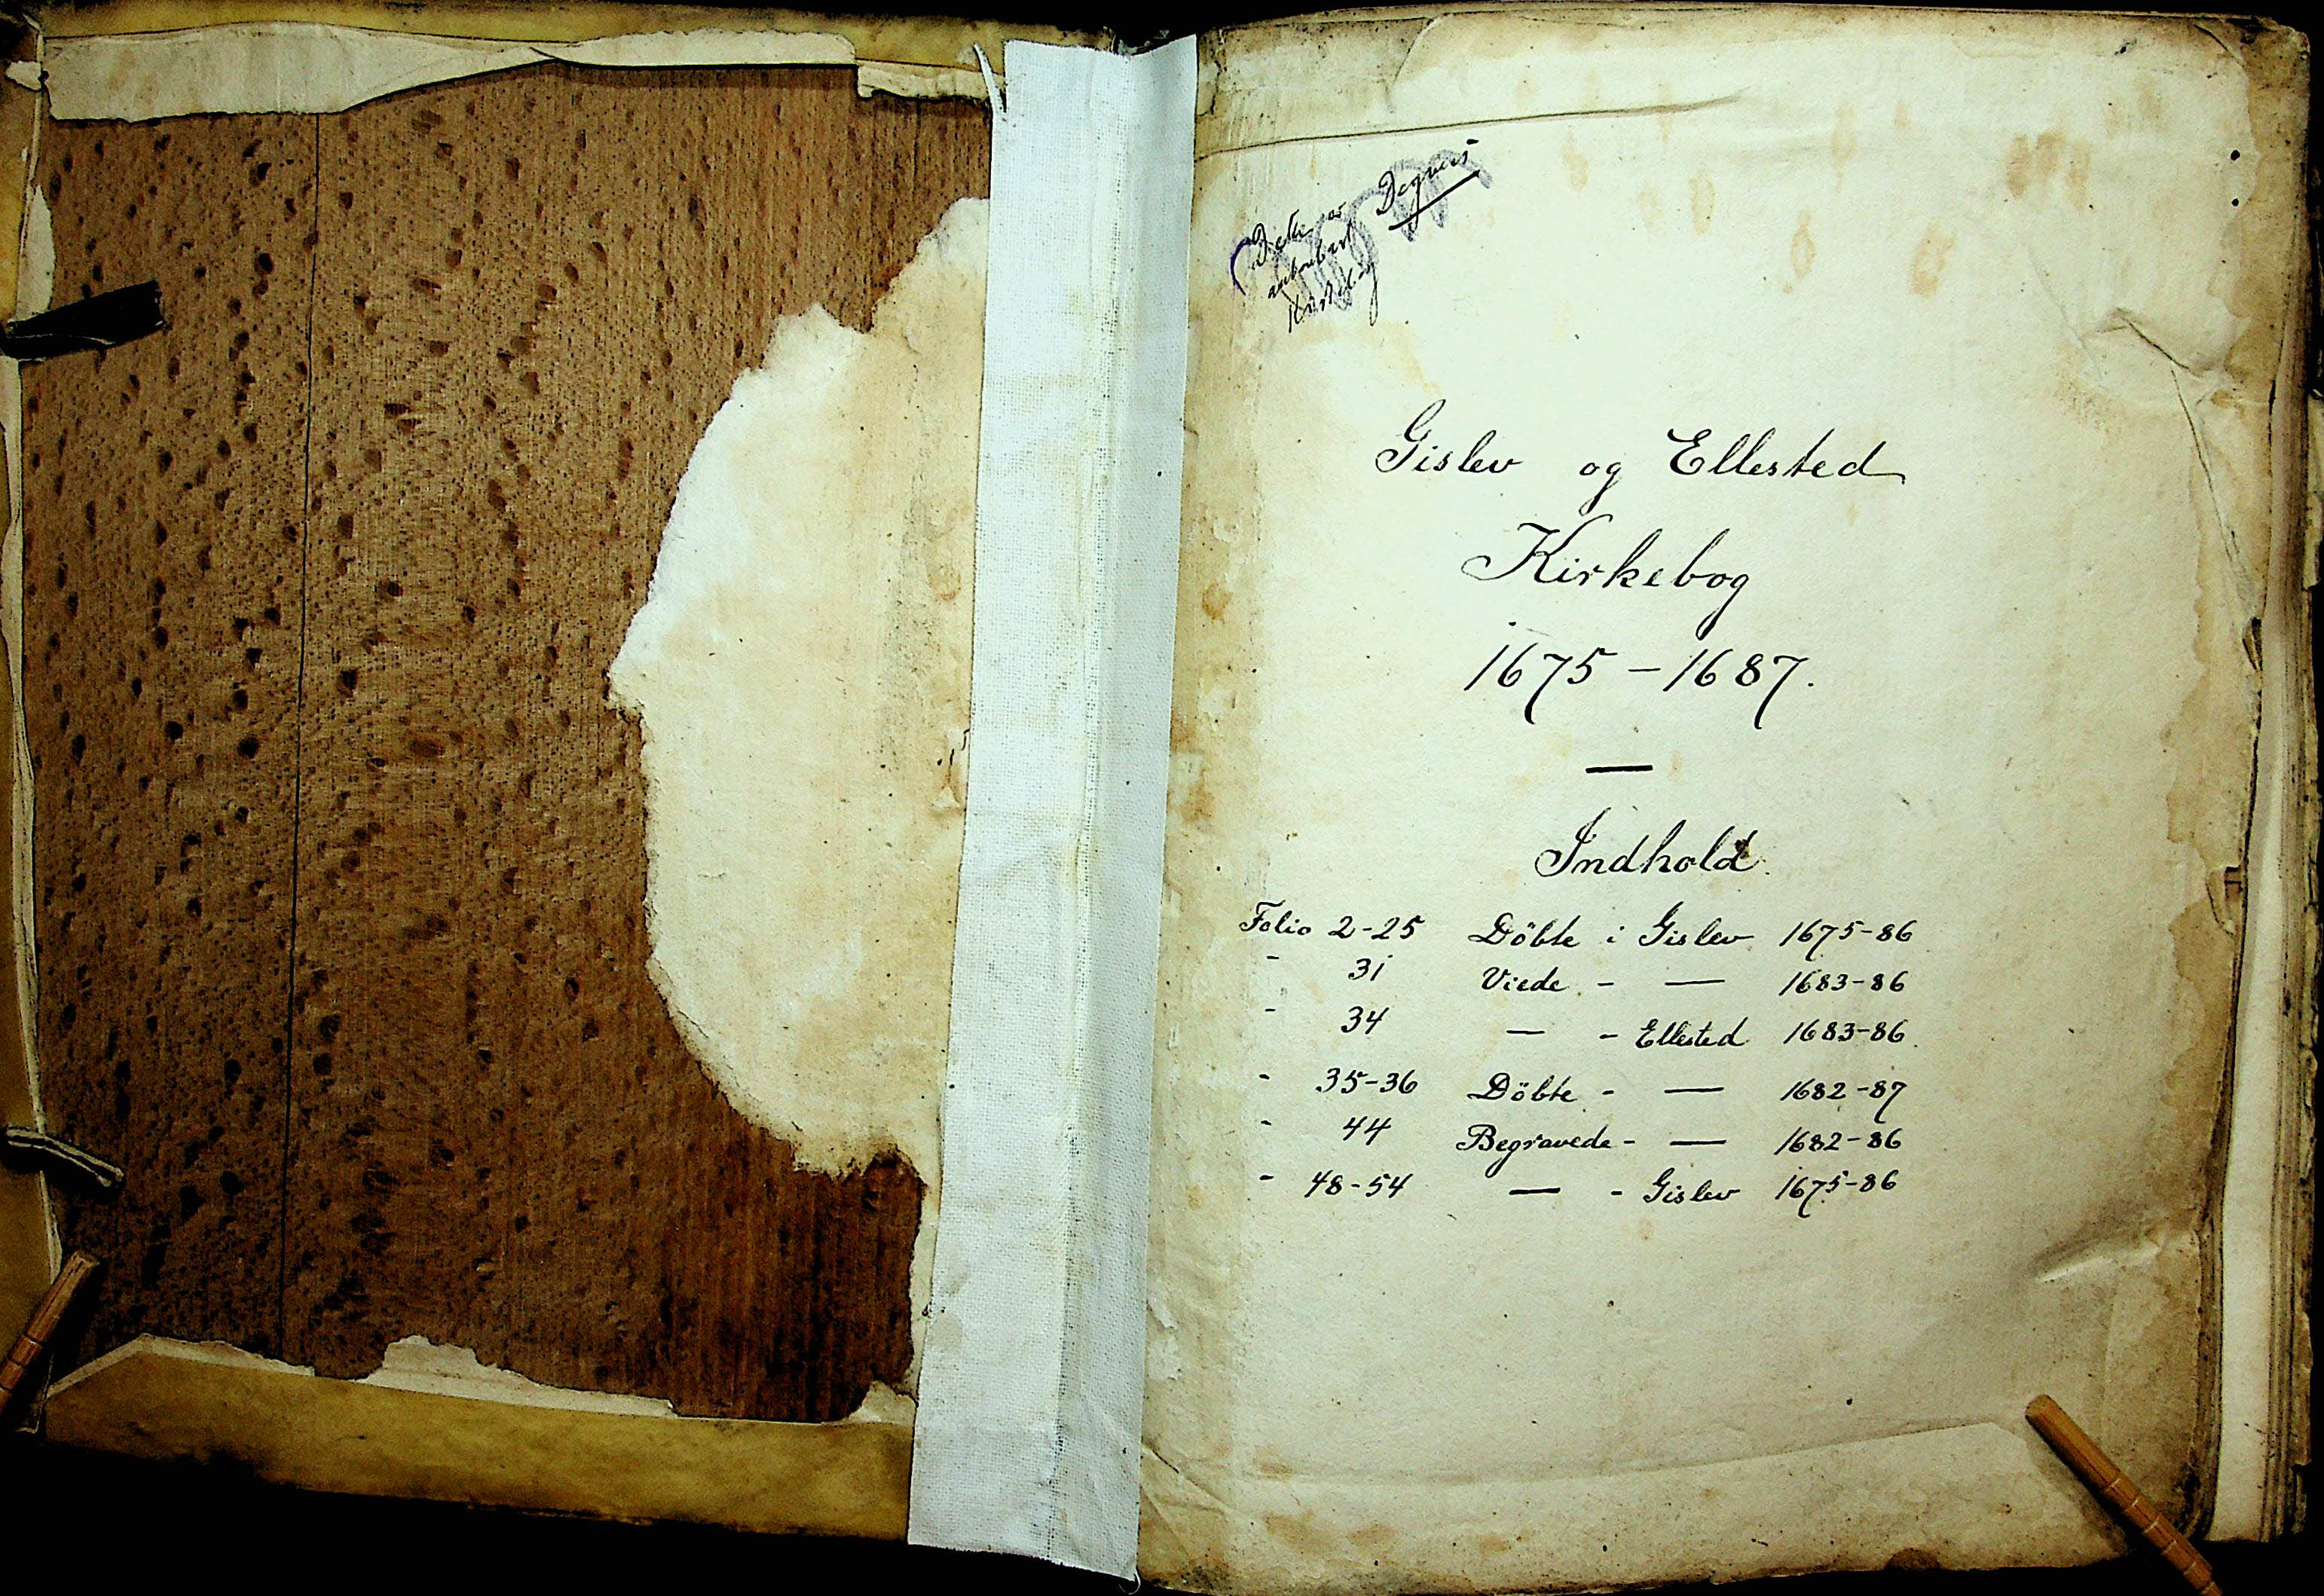Gislev og Ellested
Kirkebog
1675-1687.
Indhold
Folio 2-25 Døbte i Gislev 1675-86
- 31 Viede - - 1683-86
- 34 - - Ellested 1683-86
- 35-36 Døbte - - 1682-87
- 44 Begravede - -1682- 86
- 48-54 - - Gislev 1675-86
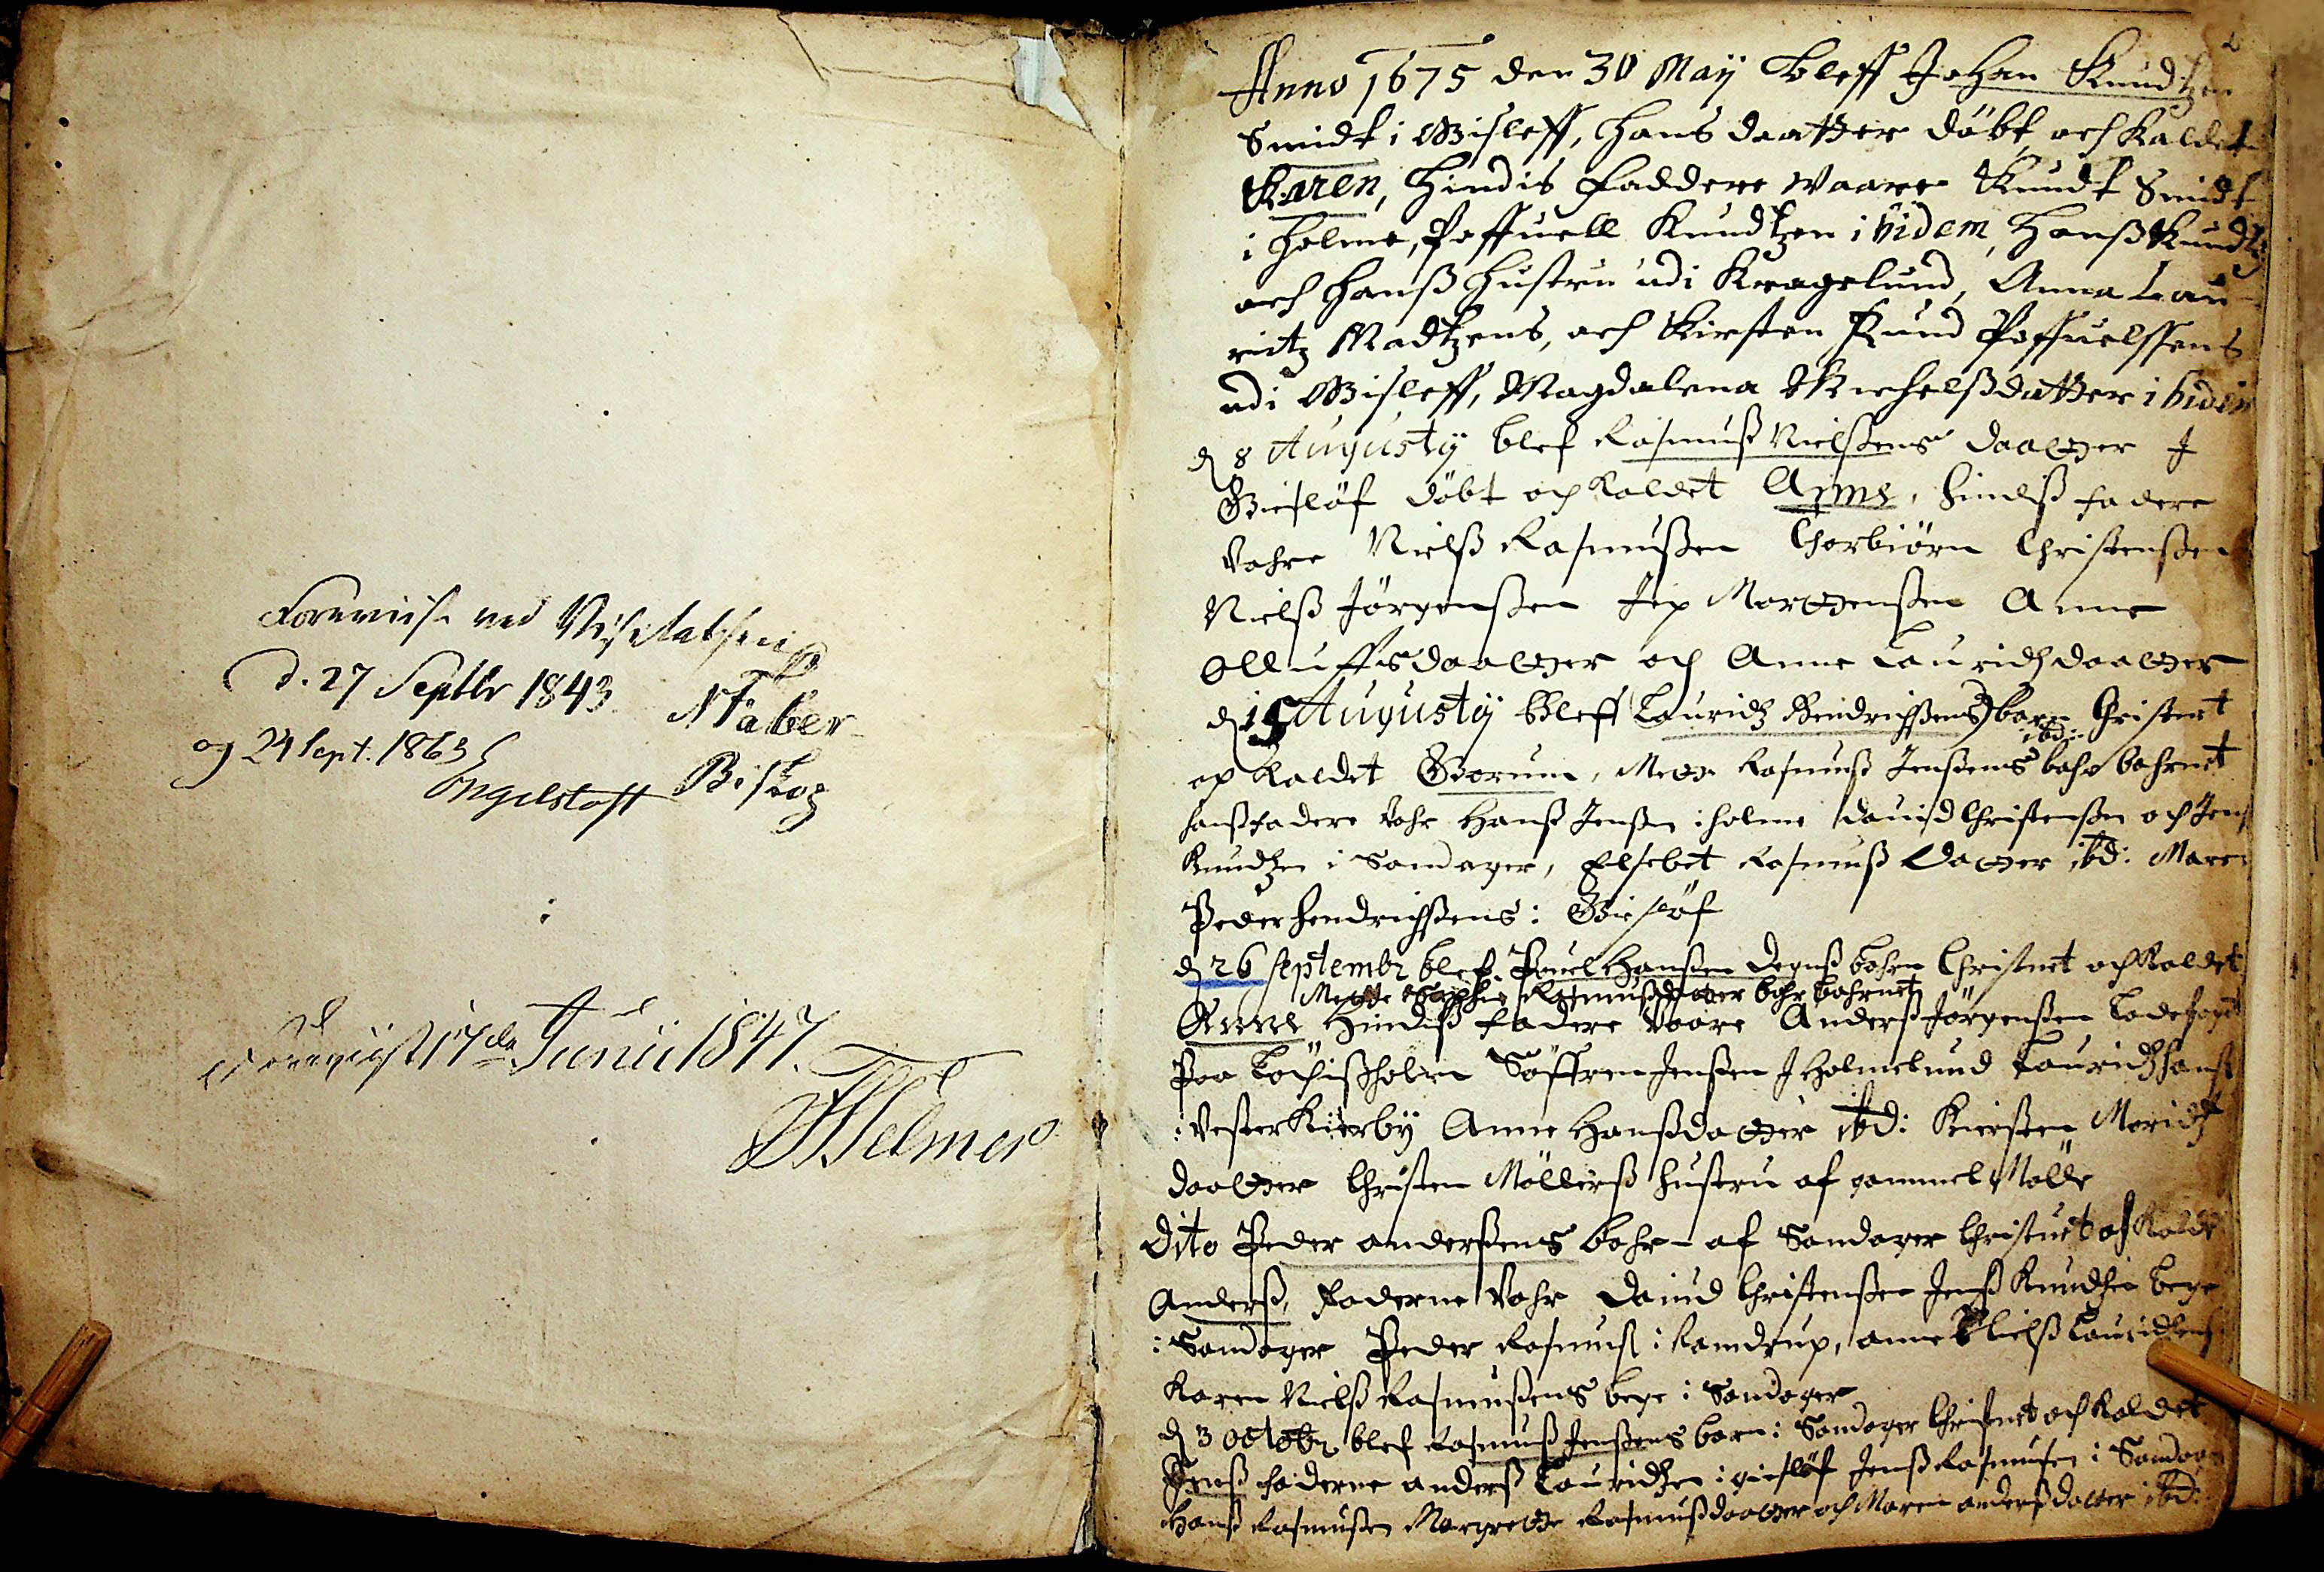Forevist ved Visitatsen
d. 27 Septbr 1843
og 24 sept. 1843
N Faber
Biskop
Engelstoff
Forevist 17de Junii 1847
##Helmer
2
Anno 1675 den 30 Maij bleff Johan Knudtzen
Smidt i Gisleff, Hans daatter døbt och kaldet
Karen, hindis Faddere vaare Knudt Smidt
i Holme, Poffuell Knudtzen ibidem, Hanss Knudtz
och Hanss Hustru udi Kragelund, Anna Lau¬
ritz Madtzens, och Kirsten Knud Poffuelssens
udi Gisleff, Magdalena ##Kiechelssdatter ibidiem
d 8 Augustij blef Rasmus Nielssens daatter I
Giesløf døbt och kaldet Anne, hindiss fadere
vahre Nielss Rasmussen, Chorbiørn Christenssen
Nielss Jørgenssen Jep Morttenssen Anne
Olluffisdaatter och Anne Lauridtzdaatter
d15 Augustij bleff Lauridtz Hendrichssens barn Christenet
ibd:
och kaldet Gorune##, Mette Rasmuss Jenssens bahr bahrnet
hans fadere vahr Hanss Jenssen i holme Dauid Christenssen och Jens
Knudzen i Sandager, Elsebet Rasmuss Datter ibd: Maren
Peder Hendrichssens i Giesløf
d 26 Septembr blef Pouel Hanssen deress bahrn Christnet och kaldet
Mette Sophie Rasmussdatter bahr bahrnet
Anne Hindiss fadere vaare Anderss Jørgenssen Ladefoget
Paa ##Løchissholm Søffren Jenssen I Holmelund Lauridtz Hanss
i Vester Kierby Anne Hanssdatter ibd: Kiersten Moridtz
daatter Christen Møllerss hustru af gammel Mølle
Dito Peder anderssens bahr af Sandager Christenet och kaldet
Anderss, Faderne vahr Daiud Christenssen Jenss Knudtzen bege
i Sandager Peder Rasmuss i Ramdrup, Anne Nielss Lauridsens
Karen Nielss Rasmussens bege i Sandager
d 3 Octobr. blef Rasmuss Jenssens barn i Sandager Christnet och kaldet
Jenss faderne Anderss Lauridtzen i Giesløf  Jenss Rasmusen i
Hanss Rasmussen Margrette Rasmussdaatter och Maren Anderssdatter ibid:.